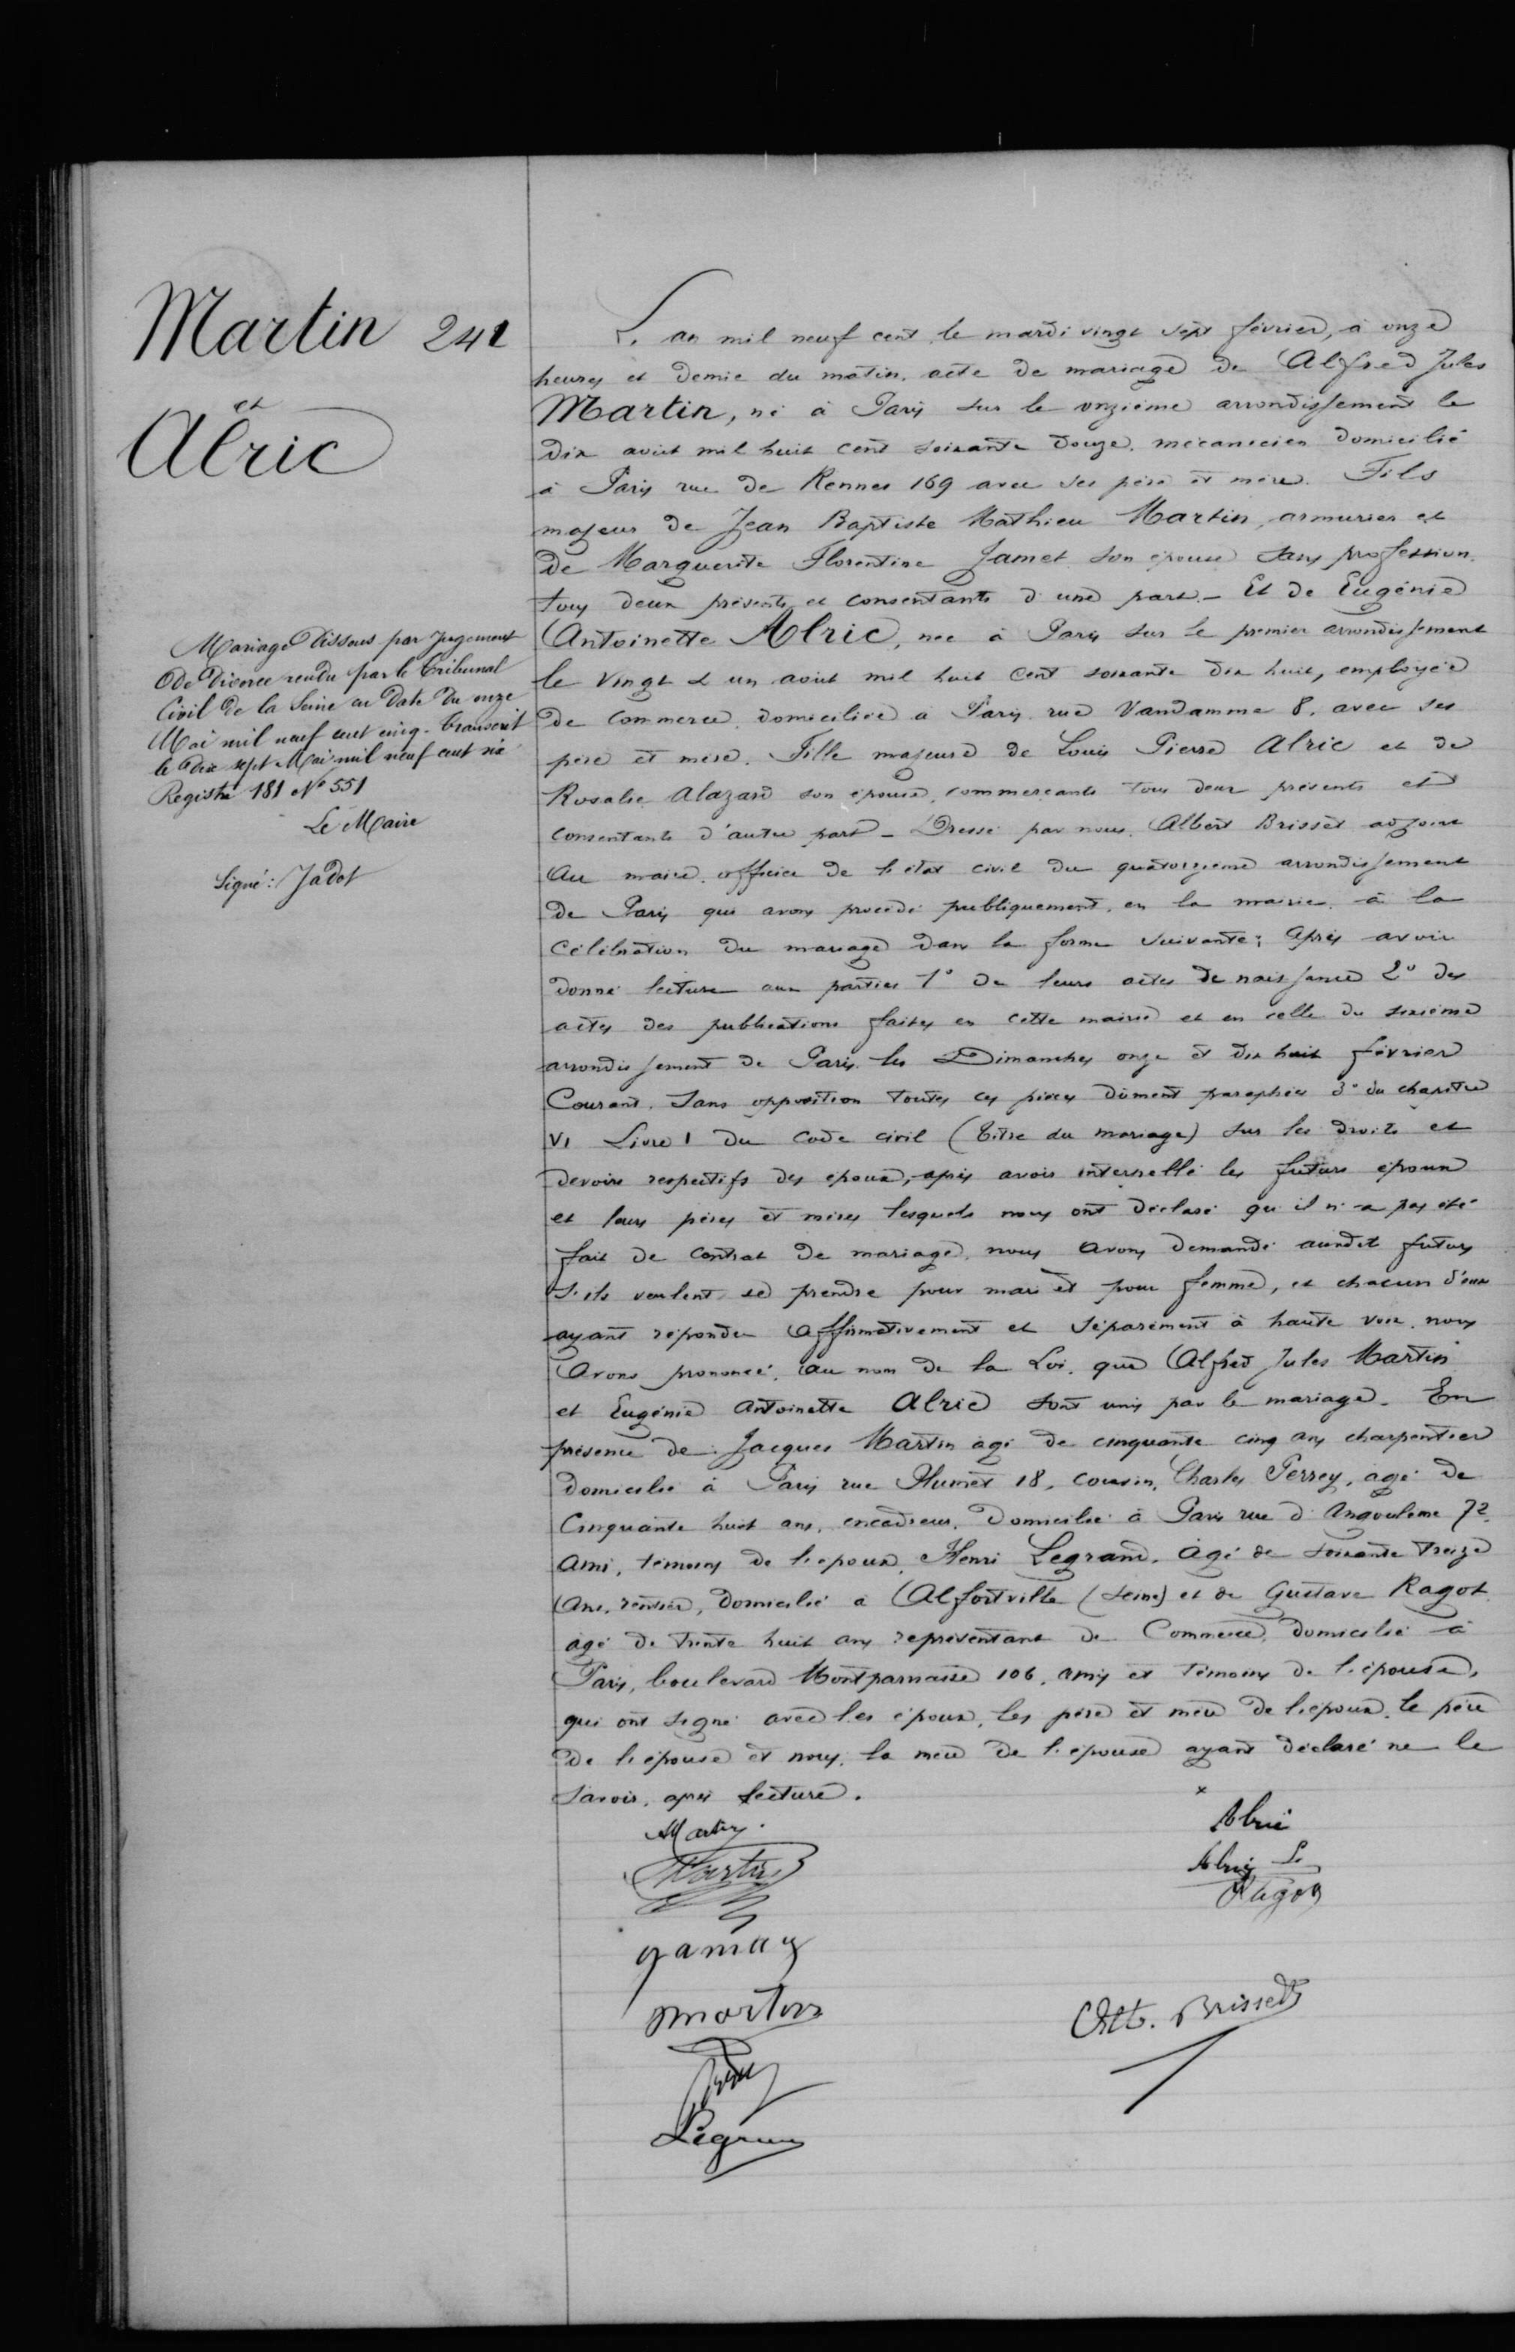Martin 241
et
Alric
L'an mil neuf cent le mardi vingt sept février, à onze
heures et demie du matin, acte de mariage de Alfred Jules
Martin, né à Paris sur le onzième arrondissement le
dix avril mil huit cent soixante douze, mécanicien domicilié
à Paris rue de Rennes, 169 avec ses père et mère Fils
majeur de Jean Baptiste Mathieu Martin, armurier et
de Marguerite Florentine Jamet son épouse sans profession
tous deux présents et consentants d'une part.-Et de Eugènie
Antoinette Alric, née à Paris sur le premier arrondissement
le vingt & un août mil huit cent soixante dix huit, employée
de commerce domiciliée à Paris rue Vaudamme 8, avec ses
père et mère. Fille majeure de Louis Pierre Alric et de
Rosalie Alazard, son épouse, commerçants tous deux présents et
consentants d'autre part -Dressé par nous Albèrt Brissès adjoint
Au maire officier de l'état civil du quatorzième arrondissement
de Paris, qui avons procédé publiquement en la mairie à la
célébration du mariage dans la forme suivante: après avoir
donné lecture aux parties 1° de leurs actes de naissance 2° des
actes de publications faites en cette maison et en celle du seizième
arrondissement de Paris les Dimanches onze et dix huit février
Courant. Sans opposition toutes les pièces dûment paraphées d'un chapitre
VI Livre I du Code civil (Titre du mariage) sur les droits et
devoirs respectifs des époux, après avoir interpellé les futurs époux
et leurs père et mères lesquels nous ont déclaré qu'il n'a pas été
fait de contrat de mariage nous avons demandé audit futurs
s'ils veulent se prendre pour mari et pour femme, et chacun d'eux
ayant répondu affirmativement et séparement à haute voix. nous
avons prononcé au nom de la Loi que Alfred Jules Martin
et Eugénie Antoinette Alric sont unis par le mariage. En
présence de Jacques Martin agé de cinquante cinq ans, charpentier
domicilié à Paris rue Hermès 18, cousin, Charles Perrey, âgé de
cinquante huit ans, encadreur, domicilié à Paris rue d'Angouleme 72
ami, témoins de l'époux, Henri Legrand, âgé de soixante treize
ans, rentier, domicilié à Alfortville (Seine) et de Gustave Ragot
âgé de trente huit ans représentant de Commerce domicilié à
Paris, boulevard Montparnasse 106, amis et témoins de l'épouse
qui ont signé avec les époux, les père et mère de l'époux le père
de l'épouse et nous, la mère de l'épouse ayant déclaré ne le
savoir, après lecture.
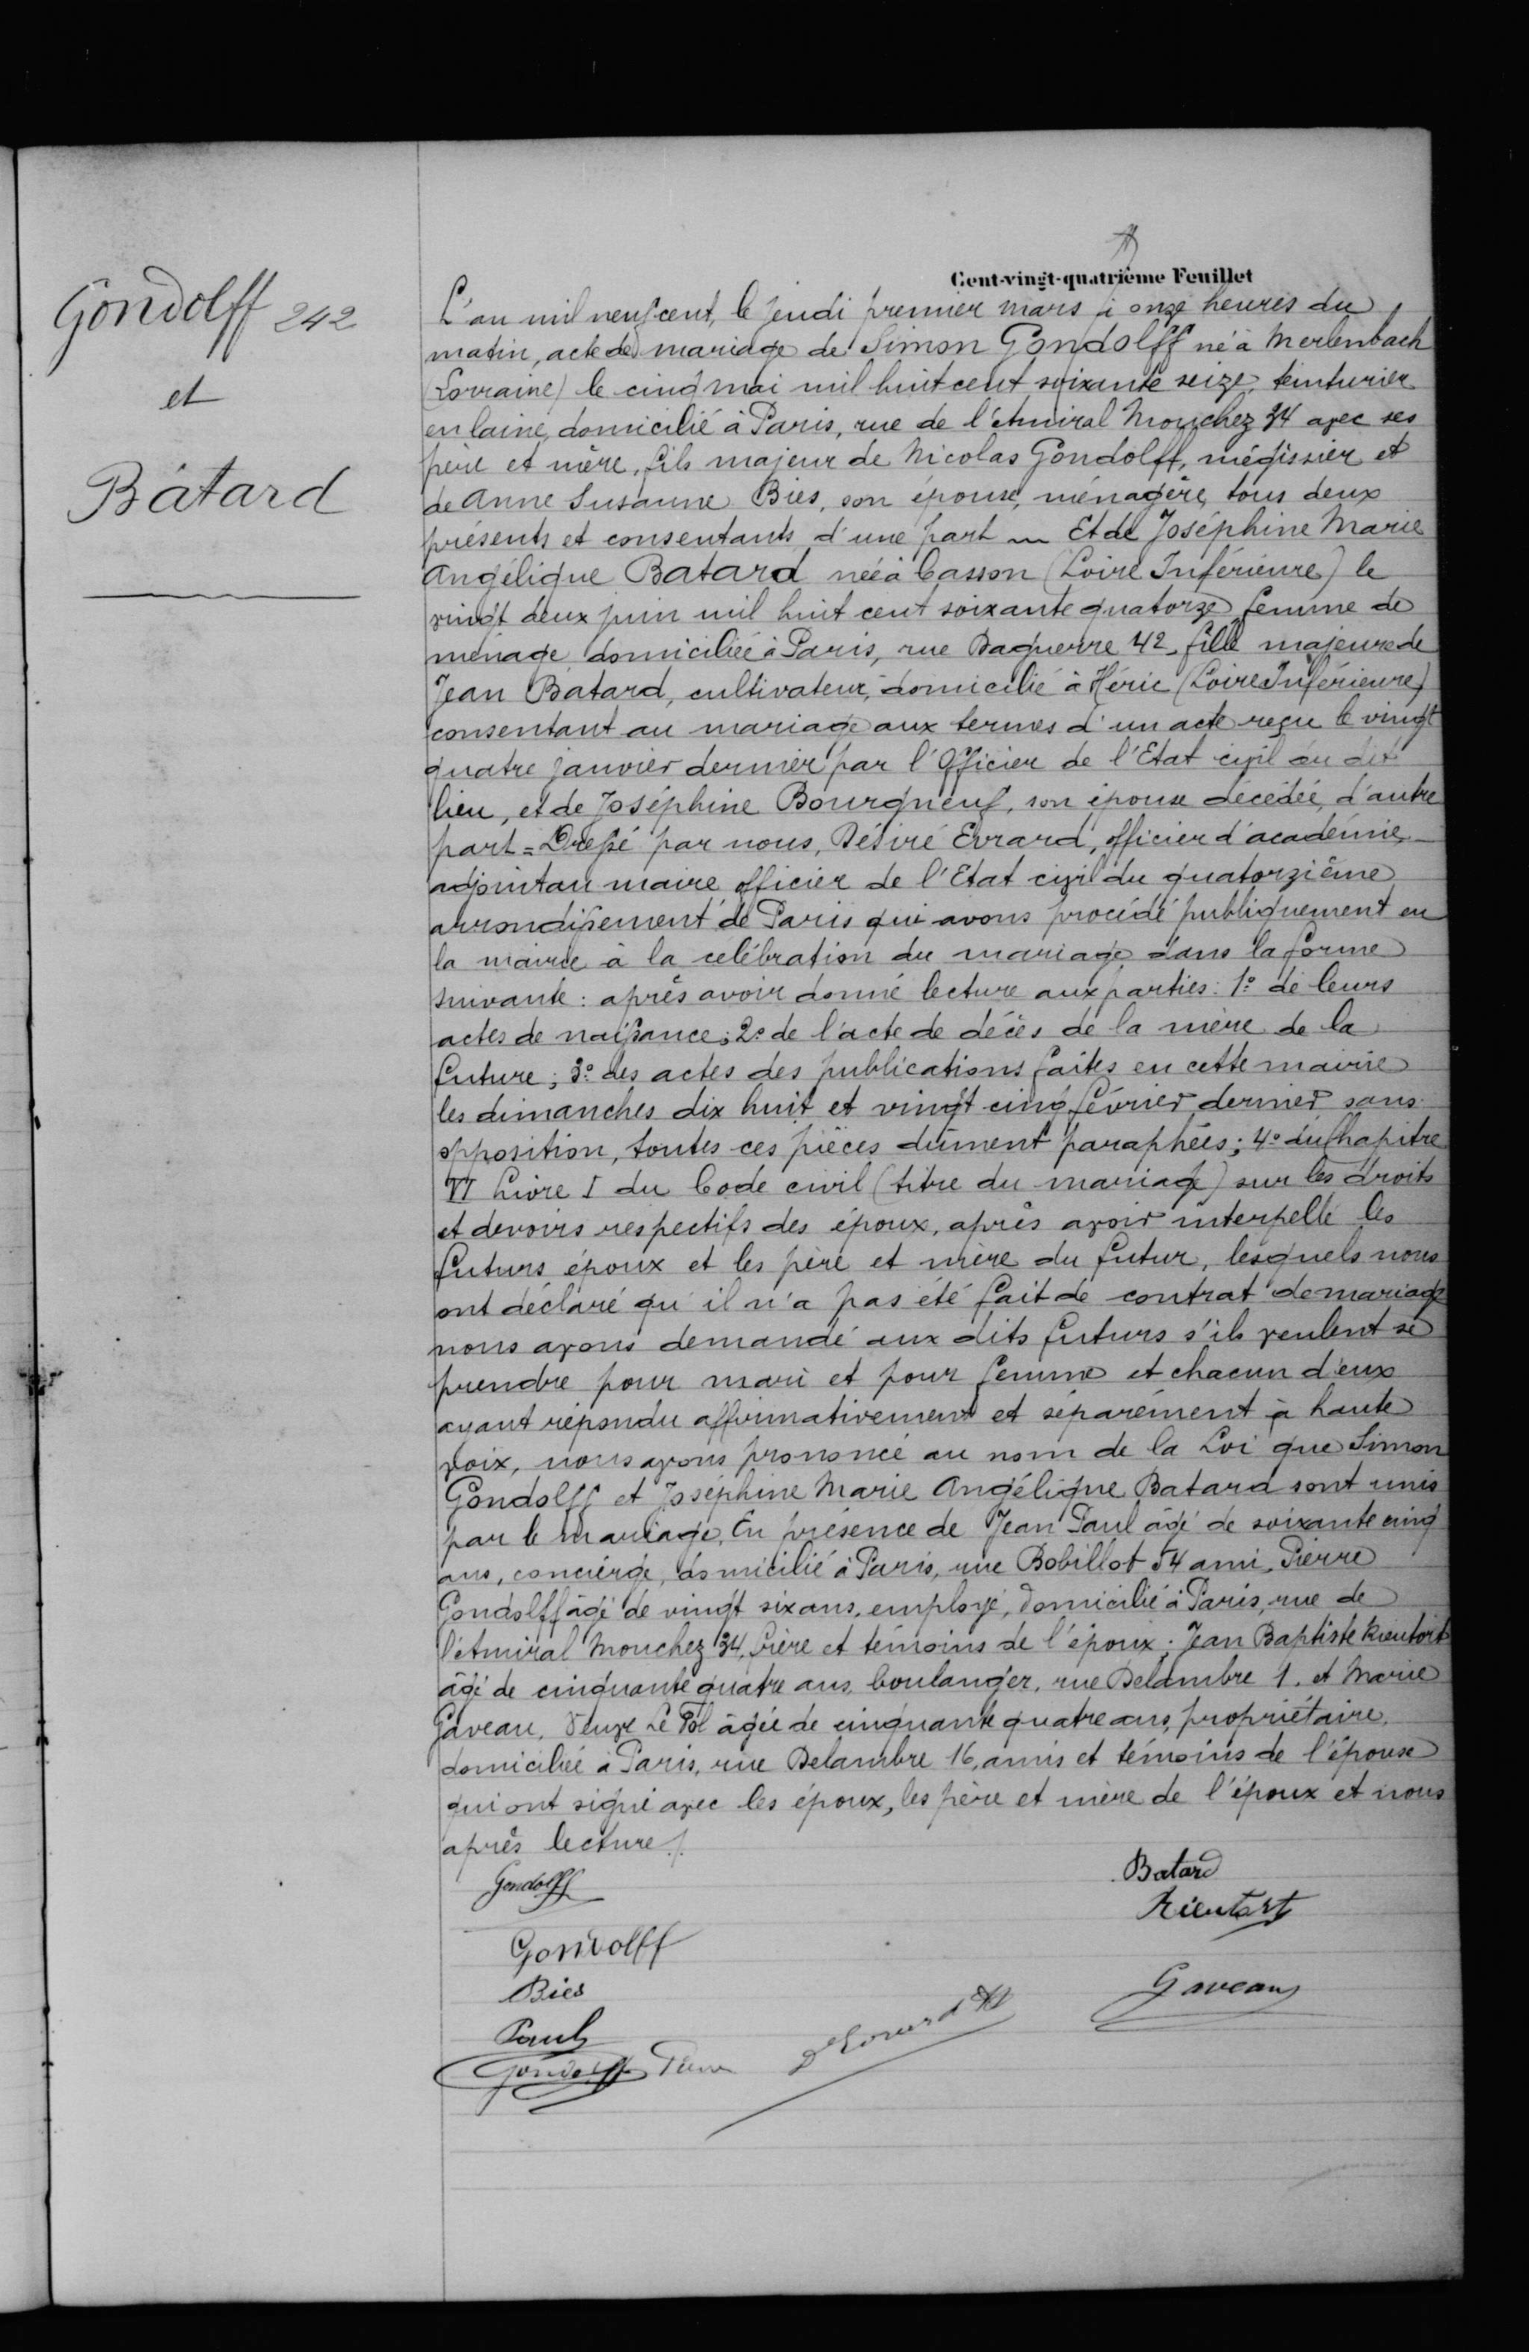Gondolff 242
et
Bâtard
L'an mil neuf cent, le jeudi premier mars à onze heures du
matin acte de mariage de Simon Gondolff né à merlenbach
(Lorraine) le cinq mai mil huit cent soixante seize teinturier
en laine, domicilié à Paris, rue de l'echiral Morichez 34, avec ses
père et mère, fils majeur de Nicolas Gondolff, mégissier et
de Anne Susanne Brés, son épouse, ménagère, tous deux
présents et consentants, d'une part -Et de Joséphine Marie
Angélique Batard née à basson (Loire Inférieure) le
vingt deux juin mil huit cent soixante quatorze, femme de
ménage domiciliée à Paris, rue Baguerre 42, fille majeure de
Jean Batard, cultivateur domicilié à Héric (Loire Inférieure)
consentant au mariage aux termes d'une acte reçu le vingt
quatre janvier dernier par l'Officier de l'Etat civil du dix
lieu, et de Joséphine Bourgneux, son épouse décédée d'autre
part. Dressé par nous, Désiré Evrard, officier d'académie
adjoint au maire officier de l'Etat civil du quatorzième
arrondissement de Paris qui avons procédé publiquement en
la mairie à la célébration du mariage dans la forme
suivante: après avoir donné lecture aux parties 1° de leurs
actes de naissance; 2° de l'acte de décès de la mère de la
future; 3° des actes des publications faits en cette mairie
les dimanches dix huit et vingt cinq février dernier sans-
opposition, toutes ces pièces dûment paraphées; 4° du chapitre
VI Livre I du Code civil (titre du mariage) sur les droits
et devoirs respectifs des époux après avoir interpellé les
futurs époux et les père et mère du futur, lesquels nous
ont déclaré qu'il n'a pas été fait de contrat de mariage
nous avons demandé aux dits futurs s'ils veulent se
prendre pour mari et pour femme et chacun d'eux
ayant répondu affirmativement et séparèment à haute
voix, nous avons prononcé au nom de la Loi que Simon
Gondolff et Joséphine Marie Angélique Batara sont unis
par le mariage en présence de Jean Paul âgé de soixante cinq
ans, concierge, domicilié à Paris, rue Bobillot 54 ami, Pierre
Gondolff, âgé de vingt six ans, employé, domicilié à Paris, rue de
l'Amiral Mouchez 34, frère et témoins de l'époux; Jean Baptiste Rentort
âgé de cinquante quatre ans, boulanger, rue Delambre 1 et Marie
Gaveau, Veuve le Fol âgée de cinquante quatre ans propriétaire
domicilié à Paris, rue Delambre 16, amis et témoins de l'épouse
qui ont signé avec les époux, les père et mère de l'époux et nous
après lecture.